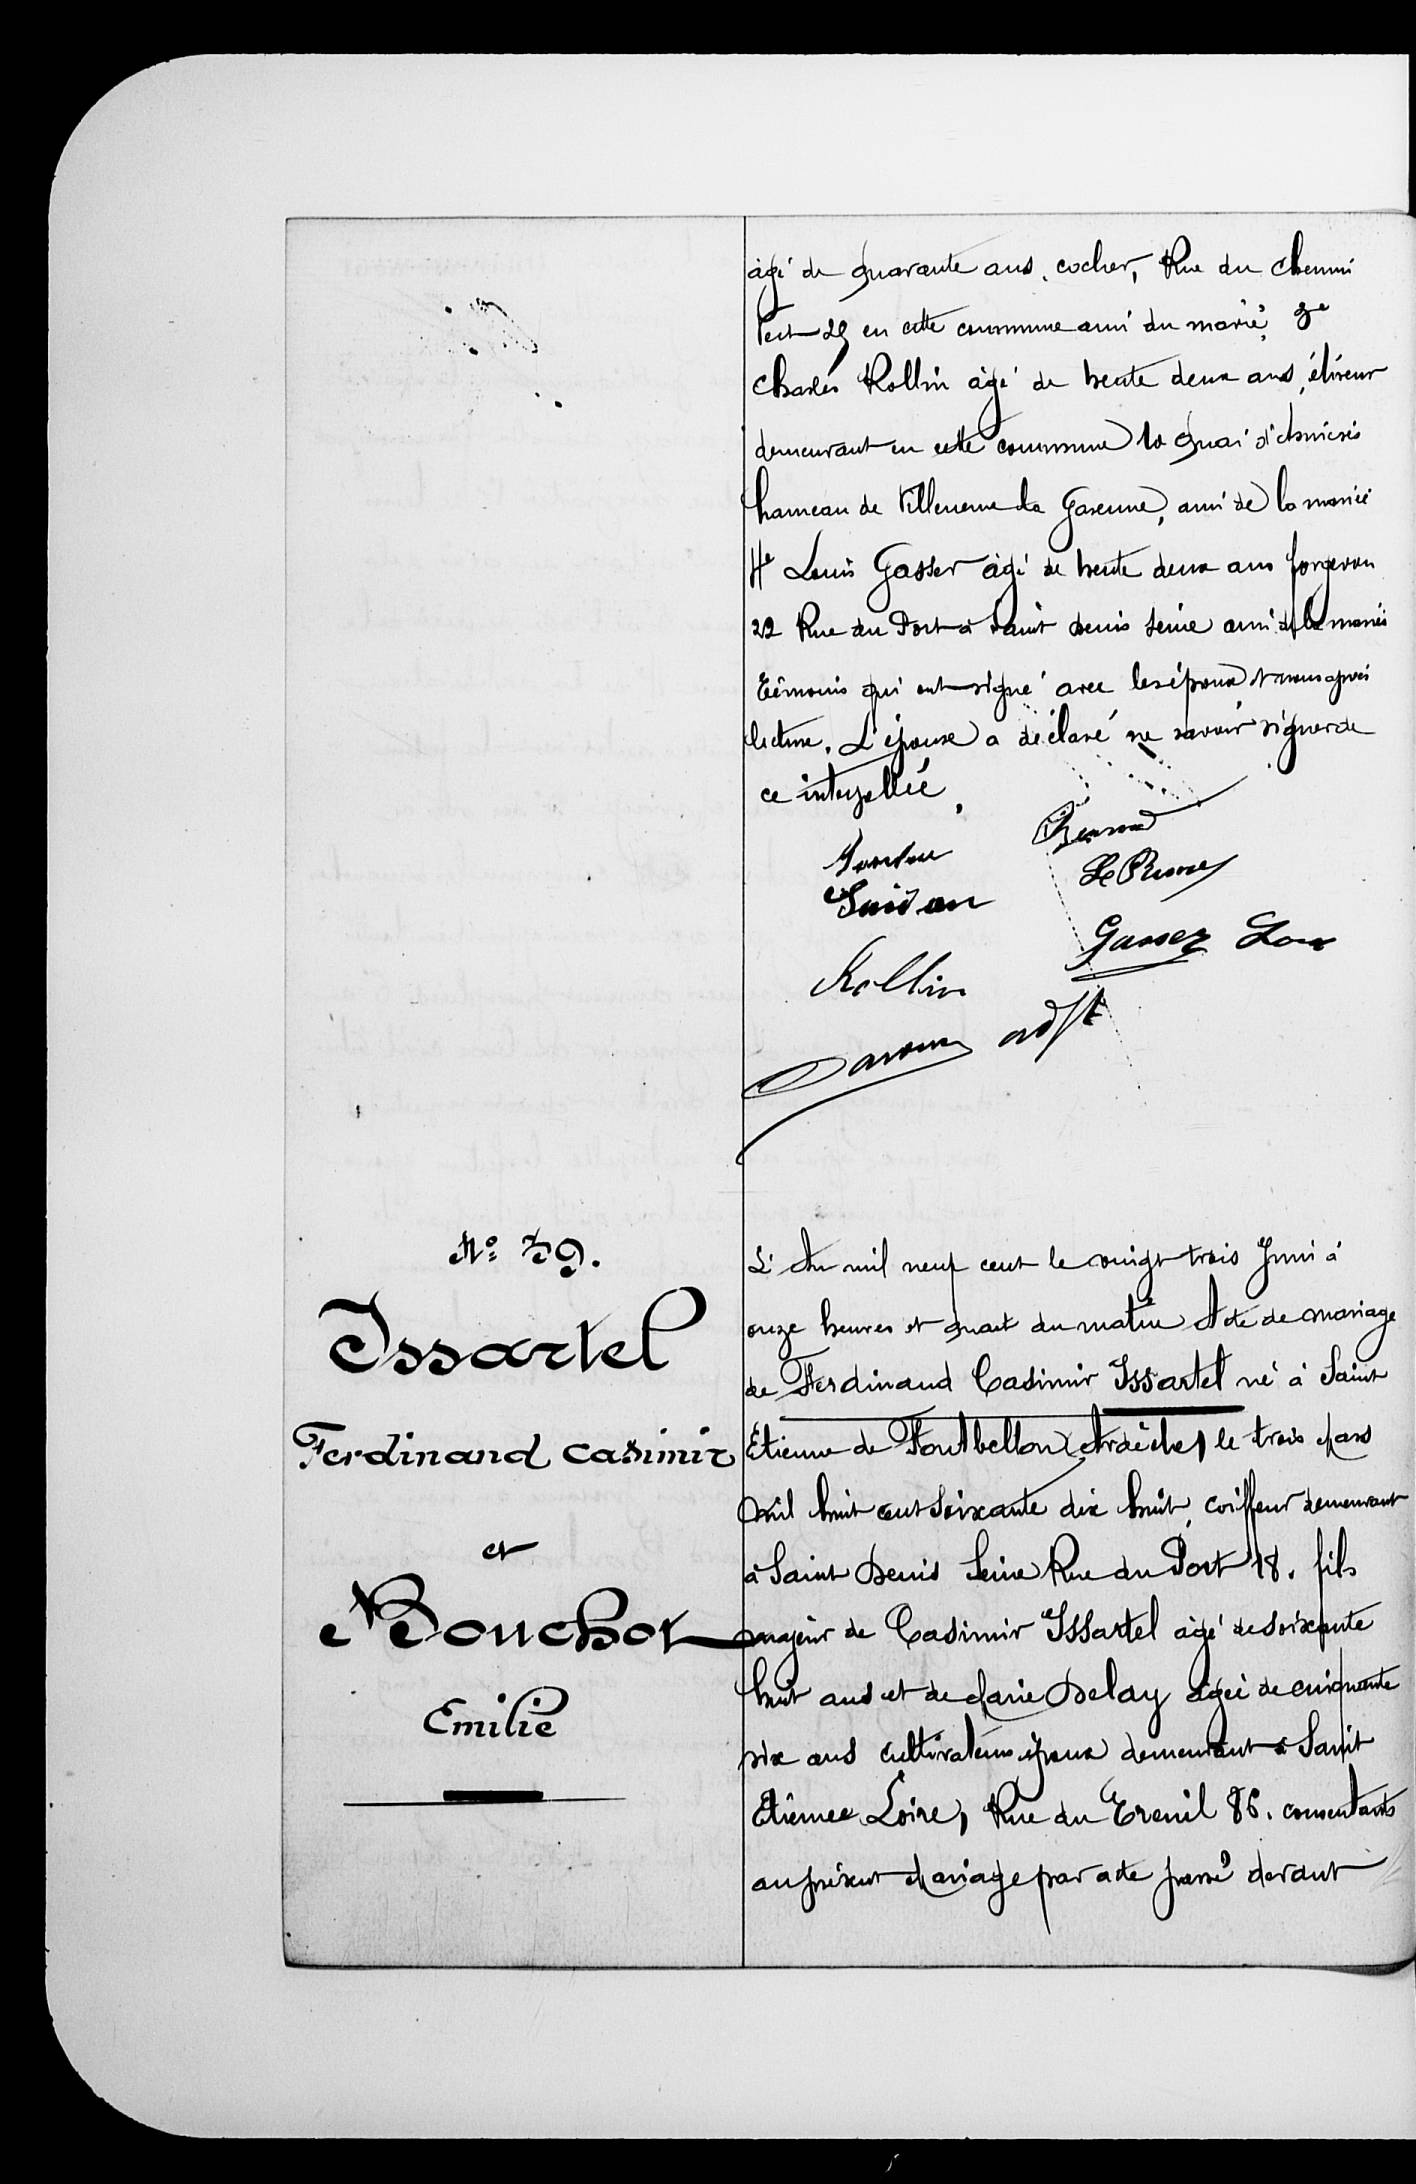âgé de quarante ans cocher, Rue du chemin
Vert 25, en cette commune ami du mairie, 3°
Charles Rollin âgé de trente deux ans, élément
demeurant en cette commune la quai d'Asnières
hameau de Villeneuve la Garenne, ami de la mariée
H Louis Gasser âgé de trente deux ans forgeron
22 Rue du Port à Saint Denis Seine ami de la mariée
témoins qui ont signé avec les époux et nous après
lecture, L'épouse a déclaré ne savoir signer de
ce interpellée.
n°39
Issartel
Ferdinanc Casimir
et
Bouchot
Emilie
L'An mil neuf cent le vingt trois juin à
onze heures et quart du matin Acte de mariage
de Ferdinand Casimir Issartel né à Saint
Etienne de Foutbellon (Ardeches) le trois mars
mil huit cent soixante dix huit coiffeur demeurant
à Saint Denis Seine Rue du Port 18, fils
majeur de Casimir Issartel âgé desoixante
huit ans et de claire Delay âgée de cinquante
six ans cultivateurs époux demeurant à Saint
Etienne Loire, Rue du Erenil 86, consentants
auprésent mariage par acte passé devant
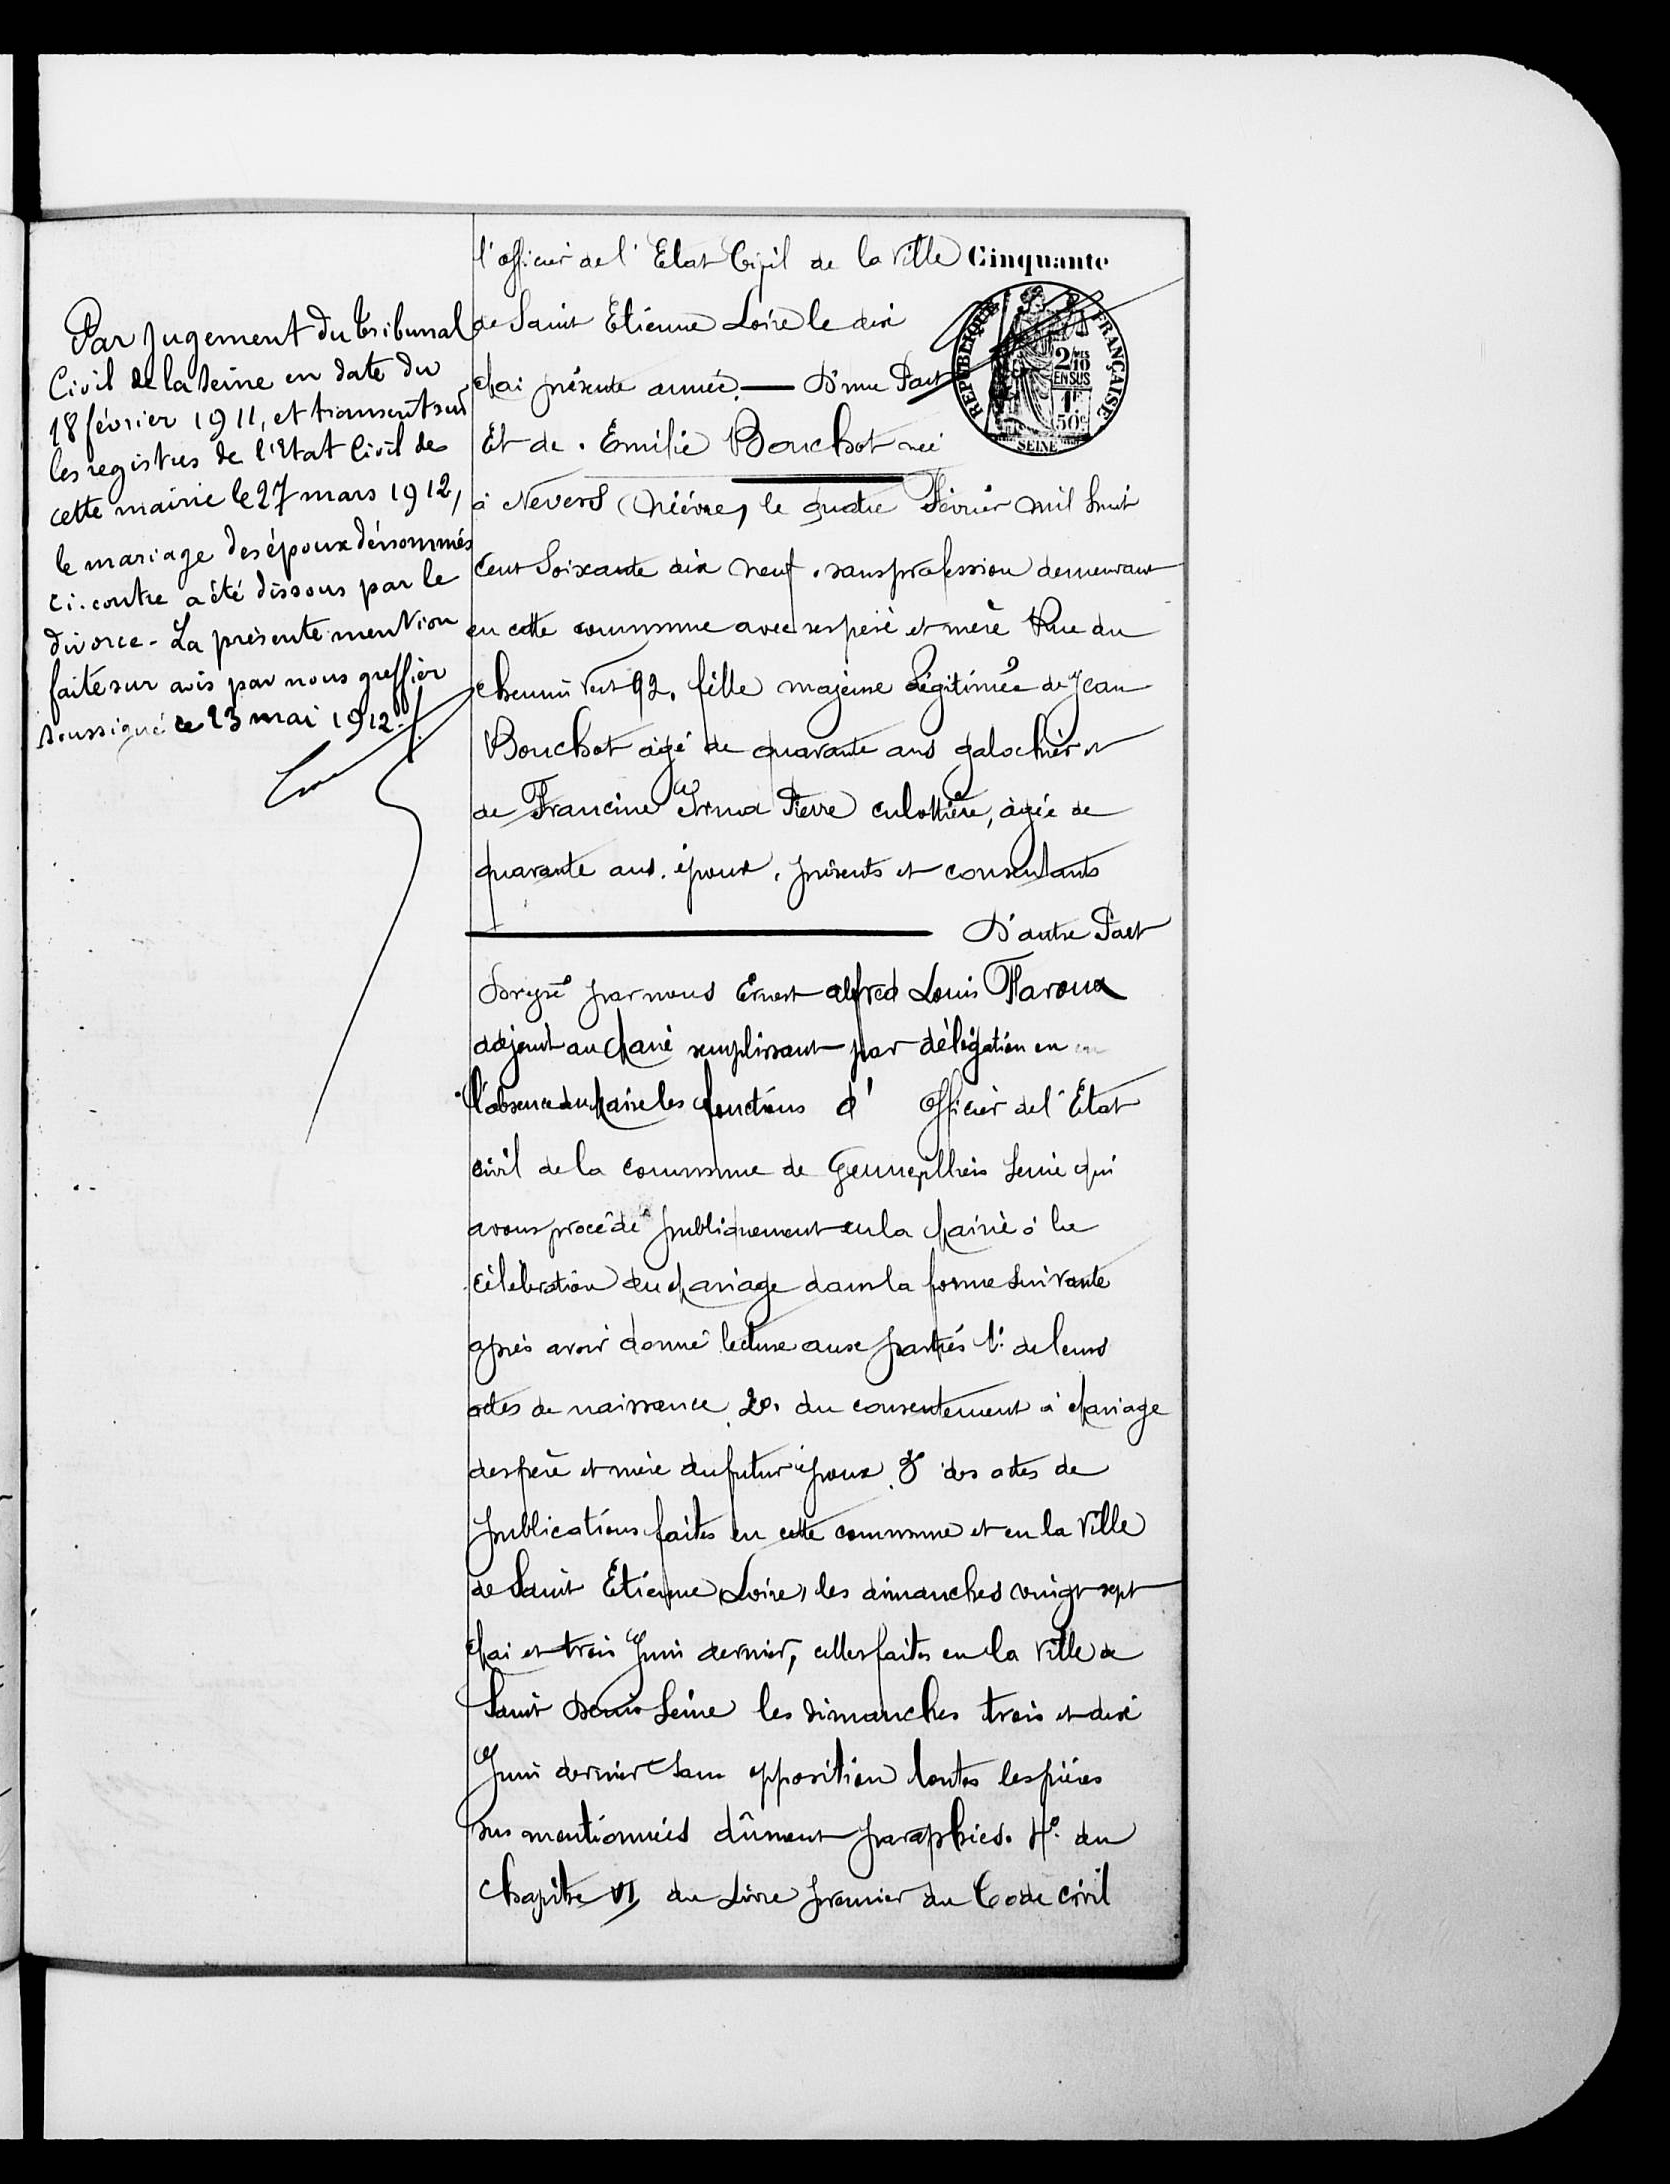l'officier de l'Etat Civil de la Ville
de Saint Etienne Loire ledix
Mai présente année -D'une Part
Et de Emilie Bouchot née
à Nevers (Nièvres) le quatre Févrir mil huit
cent soixante dix neuf, sans profession demeurant
en ctte commune avec ses père et mère Rue du
chemin vers 92, fille majeure léditimée de Jean
Bouchot âgé de quarante ans Galochier
de Francine Irma Pierre culottière, âgée de
quarante ans, épouse, présents et consentants
-D'autre Part
Signé par nous Ernest Alfred Louis Flaroux
adjoint au Maire supplissant par délégation en
l'absence du passé les fonctions d'officier del'Etat
civil de la couronne de Gennevilliers Seine qui
avons procédé publiquement en la chaine à la
célébration du Mariage dans la forme suivant
après avoir donné lecture aux parties 1° de leurs
actes de naissance, 2° du consentement à Mariage
des père et mère du futur épouse 3° des actes de
publication faites en cette commune et en la Ville
de Saint Etienne (Loire), les dimanches vingt sept
Mai en trois Juin dernier, celles faites en la Ville de
Saint Denis Seine les dimanches trois et dix
Juin dernier sans opposition toutes les parties
sus mentionnées dûment paraphées. 4° du
chapitre VI du Livre Premier du Code Civil